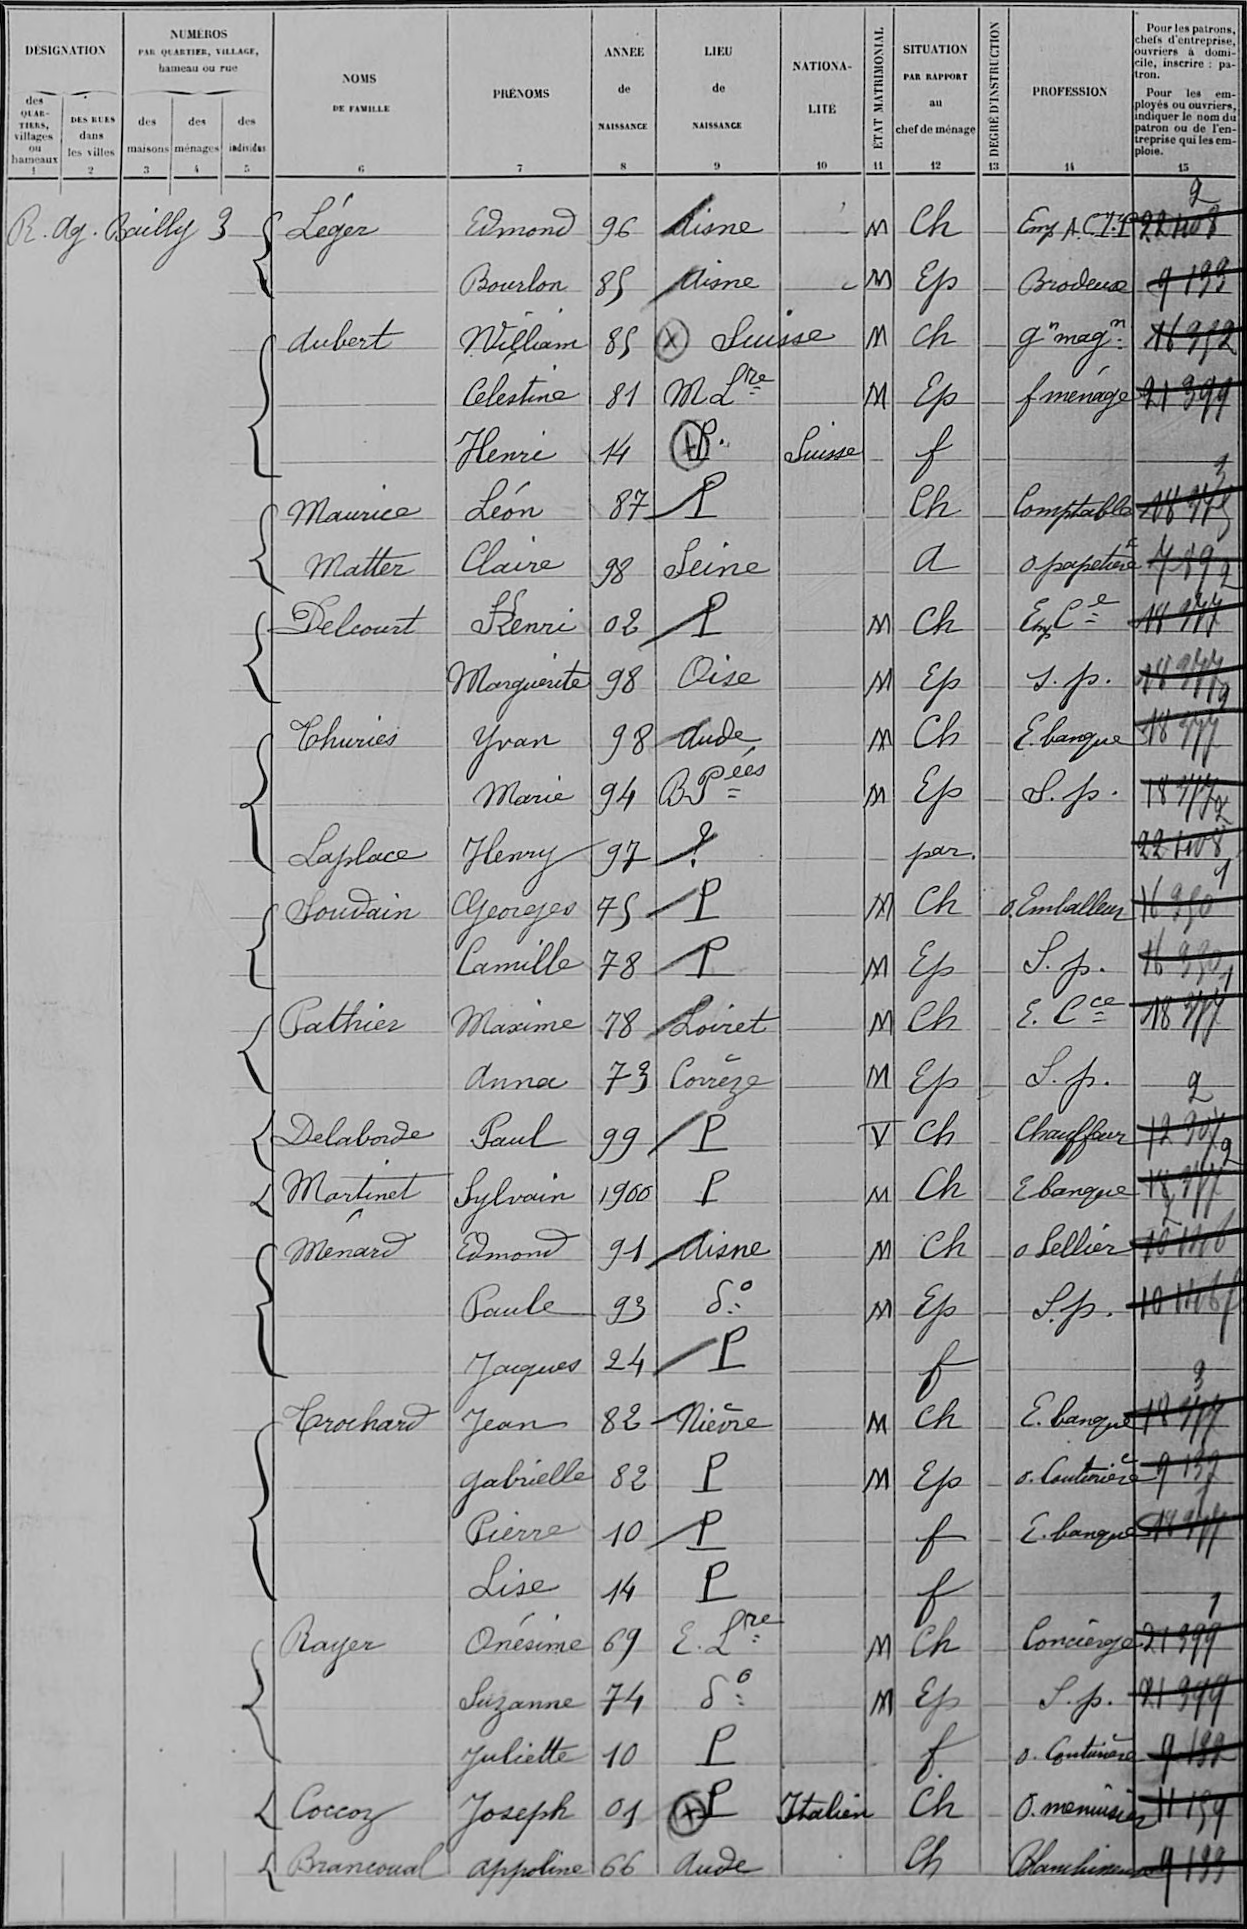Léger/Edmond/96/Aisne/¤/M/Ch/¤/Emp ACTP/22408
¤/Bourlon/85/Aisne/¤/M/Ep/¤/Brodeuse/9133
Aubert/William/85/Suisse/¤/M/Ch/¤/gn magn/16352
¤/Celestine/81/M Lre/¤/M/Ep/¤/f ménage/21399
¤/Henri/14/P/Suisse/¤/f/¤/¤/¤
Maurice/Léon/87/P/¤/¤/Ch/¤/Comptable/18375
Matter/Claire/98/Seine/¤/¤/a/¤/o papetière/7892
Delcourt/Henri/02/P/¤/M/Ch/¤/Emp Cce/18377
¤/Marguerite/98/Oise/¤/M/Ep/¤/s p /18377
Thuries/Yvan/98/Aude/¤/M/Ch/¤/E banque/18377
¤/Marie/94/B Pées/¤/M/Ep/¤/S p /18377
Laplace/Henry/97/¤/¤/¤/par/¤/¤/22408
Soudain/Georges/75/P/¤/M/Ch/¤/o Emballeur/16350
¤/Camille/78/P/¤/M/Ep/¤/S p /16350
Pathier/MAxime/78/Loiret/¤/M/Ch/¤/E Cce/18377
¤/Anna/73/Corrèze/¤/M/Ep/¤/S p /¤
Delaborde/Paul/99/P/¤/V/Ch/¤/Chauffeur/12307
Martinet/Sylvain/1900/P/¤/M/Ch/¤/E banque/18377
Menard/Edmond/91/Aisne/¤/M/Ch/¤/o Sellier/10148
¤/Paule/93/d°/¤/M/Ep/¤/Sp/10148
¤/Jacques/24/P/¤/¤/f/¤/¤/¤
Trochard/Jean/82/Nièvre/¤/M/Ch/¤/E banque/18377
¤/gabrielle/82/P/¤/M/Ep/¤/o Couturière/9132
¤/Pierre/10/P/¤/¤/f/¤/E banque/18377
¤/Lise/14/P/¤/¤/f/¤/¤/¤
Rayer/Onésine/69/E Lre/¤/M/Ch/¤/Concierge/21399
¤/Suzanne/74/d°/¤/M/Ep/¤/S p /21399
¤/Juliette/10/P/¤/¤/f/¤/o Couturière/9132
Coccoz/Joseph/01/P/Italien/¤/Ch/¤/O menuisier/11159
Brancoual/appoline/66/Aude/¤/¤/Ch/¤/Blanchisseuse/9133
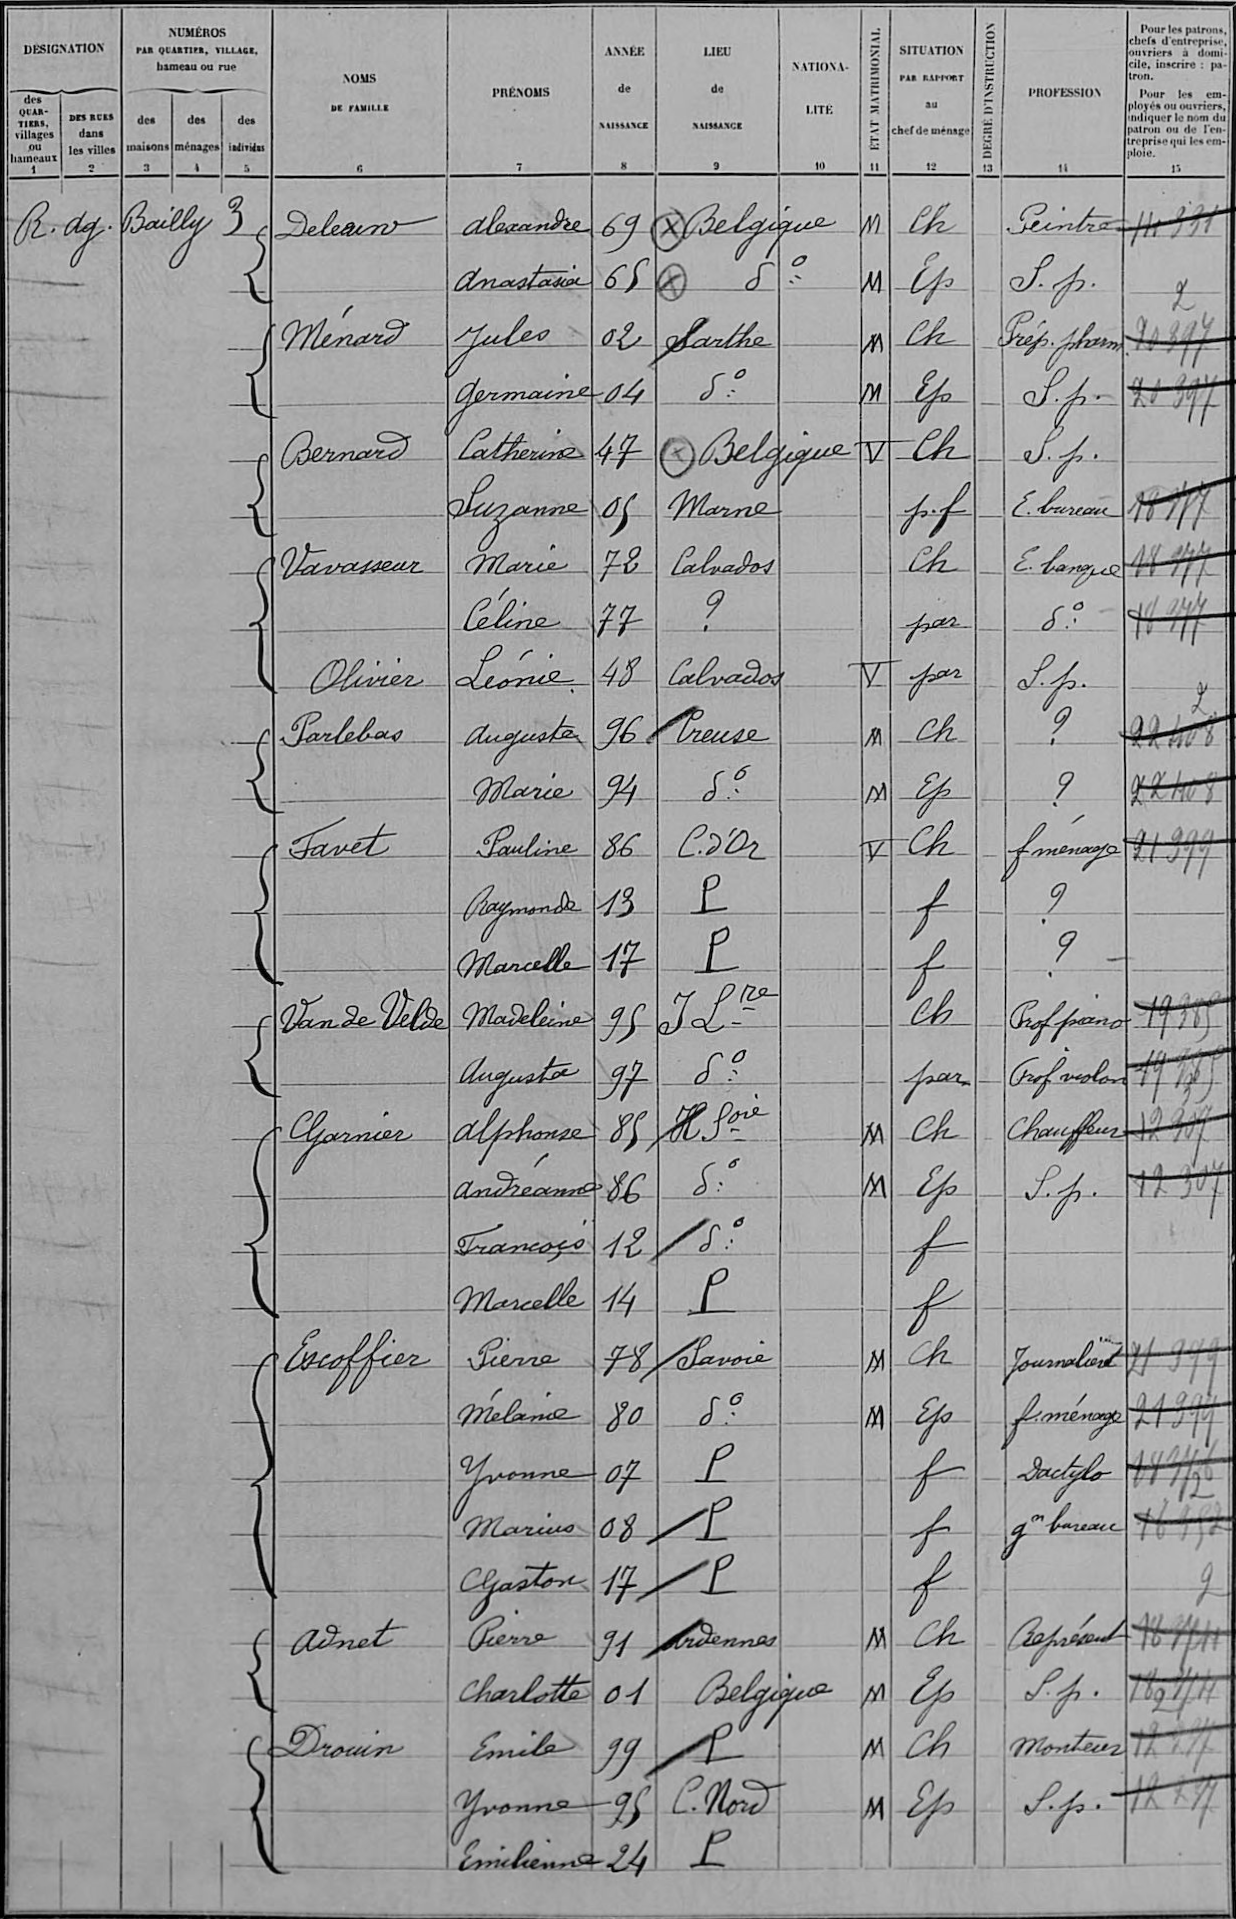Deleaine/alexandre/69/Belgique/¤/M/Ch/¤/Peintre/14331
¤/Anastasia/65/d°/¤/M/Ep/¤/S p /2
Ménard/Jules/02/Sarthe/¤/M/Ch/¤/Prép pharm/20397
¤/Germaine/04/d°/¤/M/Ep/¤/S p /20397
Bernard/Catherine/47/Belgique/¤/V/Ch/¤/S p /¤
¤/Suzanne/05/Marne/¤/¤/p f /¤/E bureau/18377
Vavasseur/Marie/72/Calvados/¤/¤/Ch/¤/E banque/18377
¤/Céline/77/¤/¤/¤/par/¤/d°/18377
Olivier/Léonie/48/Calvados/¤/V/par/¤/S p /¤
Parlebas/Auguste/96/Creuse/¤/M/Ch/¤/¤/22408
¤/Marie/94/d°/¤/M/Ep/¤/¤/22408
Favet/Pauline/86/C d'Or/¤/V/Ch/¤/f ménage/21399
¤/Raymonde/13/P/¤/¤/f/¤/¤/¤
¤/Marcelle/17/P/¤/¤/f/¤/¤/¤
Van de Velde/Madeleine/95/I Lre/¤/¤/Ch/¤/Prof piano/19385
¤/Augusta/97/d°/¤/¤/par/¤/Prof violon/19385
Garnier/Alphonse/85/H Loire/¤/M/Ch/¤/Chauffeur/12307
¤/andréanne/86/d°/¤/M/Ep/¤/S p /12307
¤/François/12/d°/¤/¤/f/¤/¤/¤
¤/Marcelle/1/P/¤/¤/f/¤/¤/¤
Escoffier/Pierre/78/Savoie/¤/M/Ch/¤/Journalière/21399
¤/Mélanie/80/d°/¤/M/Ep/¤/f méange/21399
¤/Yvonne/07/P/¤/¤/f/¤/Dactylo/18376
¤/Marius/08/P/¤/¤/f/¤/gn bureau/18352
¤/Gaston/17/P/¤/¤/f/¤/¤/2
Adnet/Pierre/91/ardennes/¤/M/Ch/¤/Représent/18374
¤/Charlotte/01/Belgique/¤/M/Ep/¤/S p /18374
Drouin/Emile/99/P/¤/M/Ch/¤/Monteur/12237
¤/Yvonne/95/C Nord/¤/M/Ep/¤/S p /12237
¤/Emilienne/24/P/¤/¤/¤/¤/¤/¤